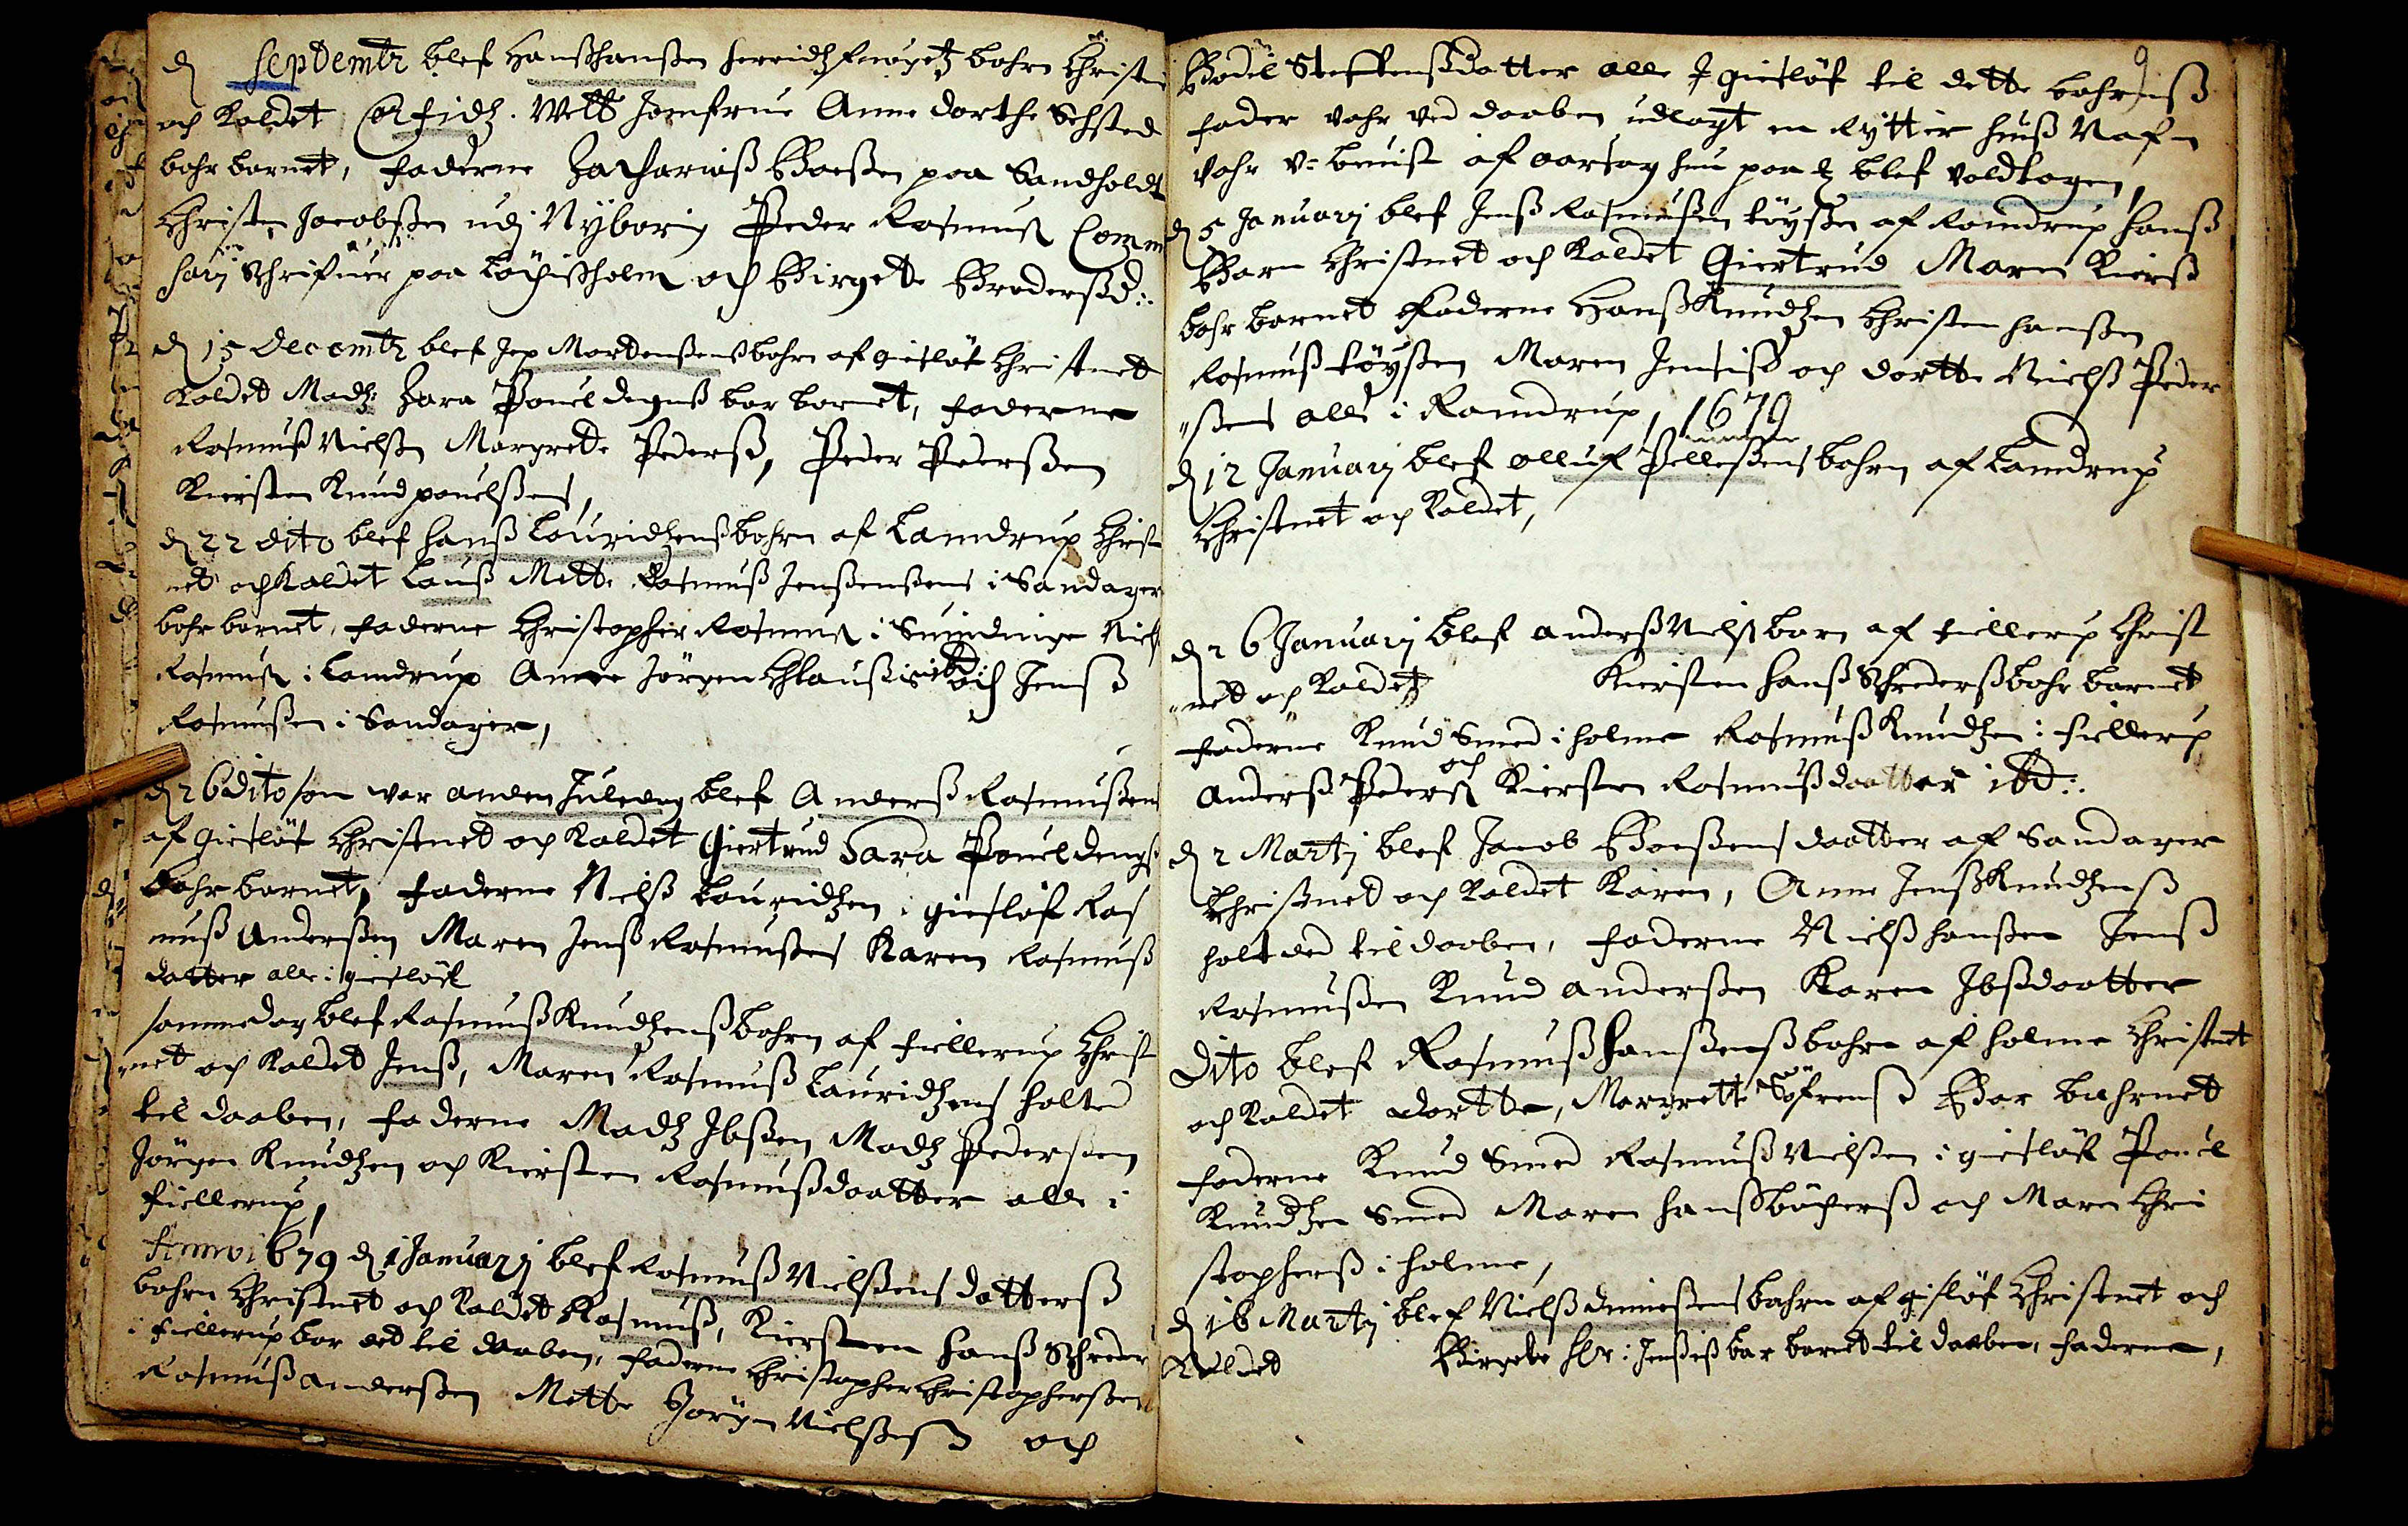d Septembr blef Hanss Hanssen Herridtz fuogetz bahrn Christn
och kaldet Corfidtz.  Well Jomfrue Anne Dorthe Sehsted
bahr barnet, Faderne Zachariass Boessen paa Sandholdt
Christen Jacobssen udi Nyborg Peder Rasmus Comm
harij## schrifuer paa Løchissholm och Birgette Brodersd:.
d 15 Decemtr blef Jep Mortenssenss bahrn af giesløf Christnet
kaldet Madtz Zara Pouel degnss bar barnet, Faderne
Rasmusss Nielss Margrete Pederss, Peder Pederssen
Kiersten Knud pouelssens
d 22 dito blef Hanss Louridtzenss bahrn af Lamdrup Christ¬
net och kaldet Lauss Mette Rasmuss Jenssenssenss i Sandager
bahr barnet, Faderne Christopher Rasmuss i Suindinge Niels 
Rasmuss i Lamdrup Anne Jørgen Claussis ibd: Jenss
Rasmussen i Sandager,
d 26 dito som var anden Juledag blef Anders Rasmussens
af Giesløf Christnet og kaldet Giertrud Sara Pouel Dengs
bahr barnet, Faderne Nielss Lauridtzen i giesløf Ras
muss Anderssen Maren Jenss Rasmussens Karen Rasmuss
datter alie i giesløf
samme dag blef Rasmuss Knudtzenss bahrn af fiellerup Christ¬
= net och kaldet Jenss, Maren Rasmuss Lauridtzens holte
til daaben, Faderne Madtz Ibssen Madtz Pederssen
Jørgen Knudtzen och Kiersten Rasmussdaatter alle i
Fiellerup
Anno 1679 d 1 Jaruarij blef Rasmuss Nielssens datterss
bahrn Christnet og kaldet Rasmuss Kiersten Hanss Schreder
i Fiellerup bar det til Daaben, Faderene Christopher Christophersen
Rasmuss Anderssen Mette Jørgen Nielssess och
9.
Bodil Steffenssdatter alle I Giesløf til dette bahrnss
fader vahr ved daaben udlagt en Rytter huess Nafn
vohr v=beuist af aarsag hun paa ## blef voldagen
d 5 Januarij blef Jenss Rasmussen tøyssen af Ramdrup Hanss
Barn Christnet och kaldet Giertrud Maren Kierss
bahr barnet Faderne Hanss Knudtzen Christen Hanssen
Rasmuss tøyssen Maren Jensiss och Dortte Nielss Peder
= sens alle i Ramdrup, 1679
D 12 Januarj bleff Olluff Pellersens bahrn af Lauedrup
Christnet og kaldet,
d 26 Januarij bleff Anderss Niels barn af Fiellerp Christ
= net och kaldet
Kiersten Hanss Schrederss bahr barnet
Faderne Knud Smed i Holme Rasmuss Knudtzen i Fiellerup
och
Anderss Peders Kiersten Rasmusdaatter ibd:.
d 2 Martij blef Jacob Boessens daatter af Sandager
Christned och kaldet Karen, Anne Jenss Knudtzenss
holt det til daaben, Faderne Nielss Hanssen, Jenss
Rasmssen Knud Anderssen Karen Ibssdaatter
Dito blef Rasmuss Hanssenss bahrn af Holme Christenet
och kaldet Dortte, Margrette Søfrenss Bar bahrnet
Faderne Knud Smed Rasmuss Nielssen i giesløf Pouel
Knudtzen Smed Maren Hanss bøcherss och Maren Chri
stopherss i holme
d 16 Martij blef Nielss dinnessens bahrn af gisløf Christenet och
kaldet
Birgete hlr: Jenssess bar barnet til Daaben, Faderne,
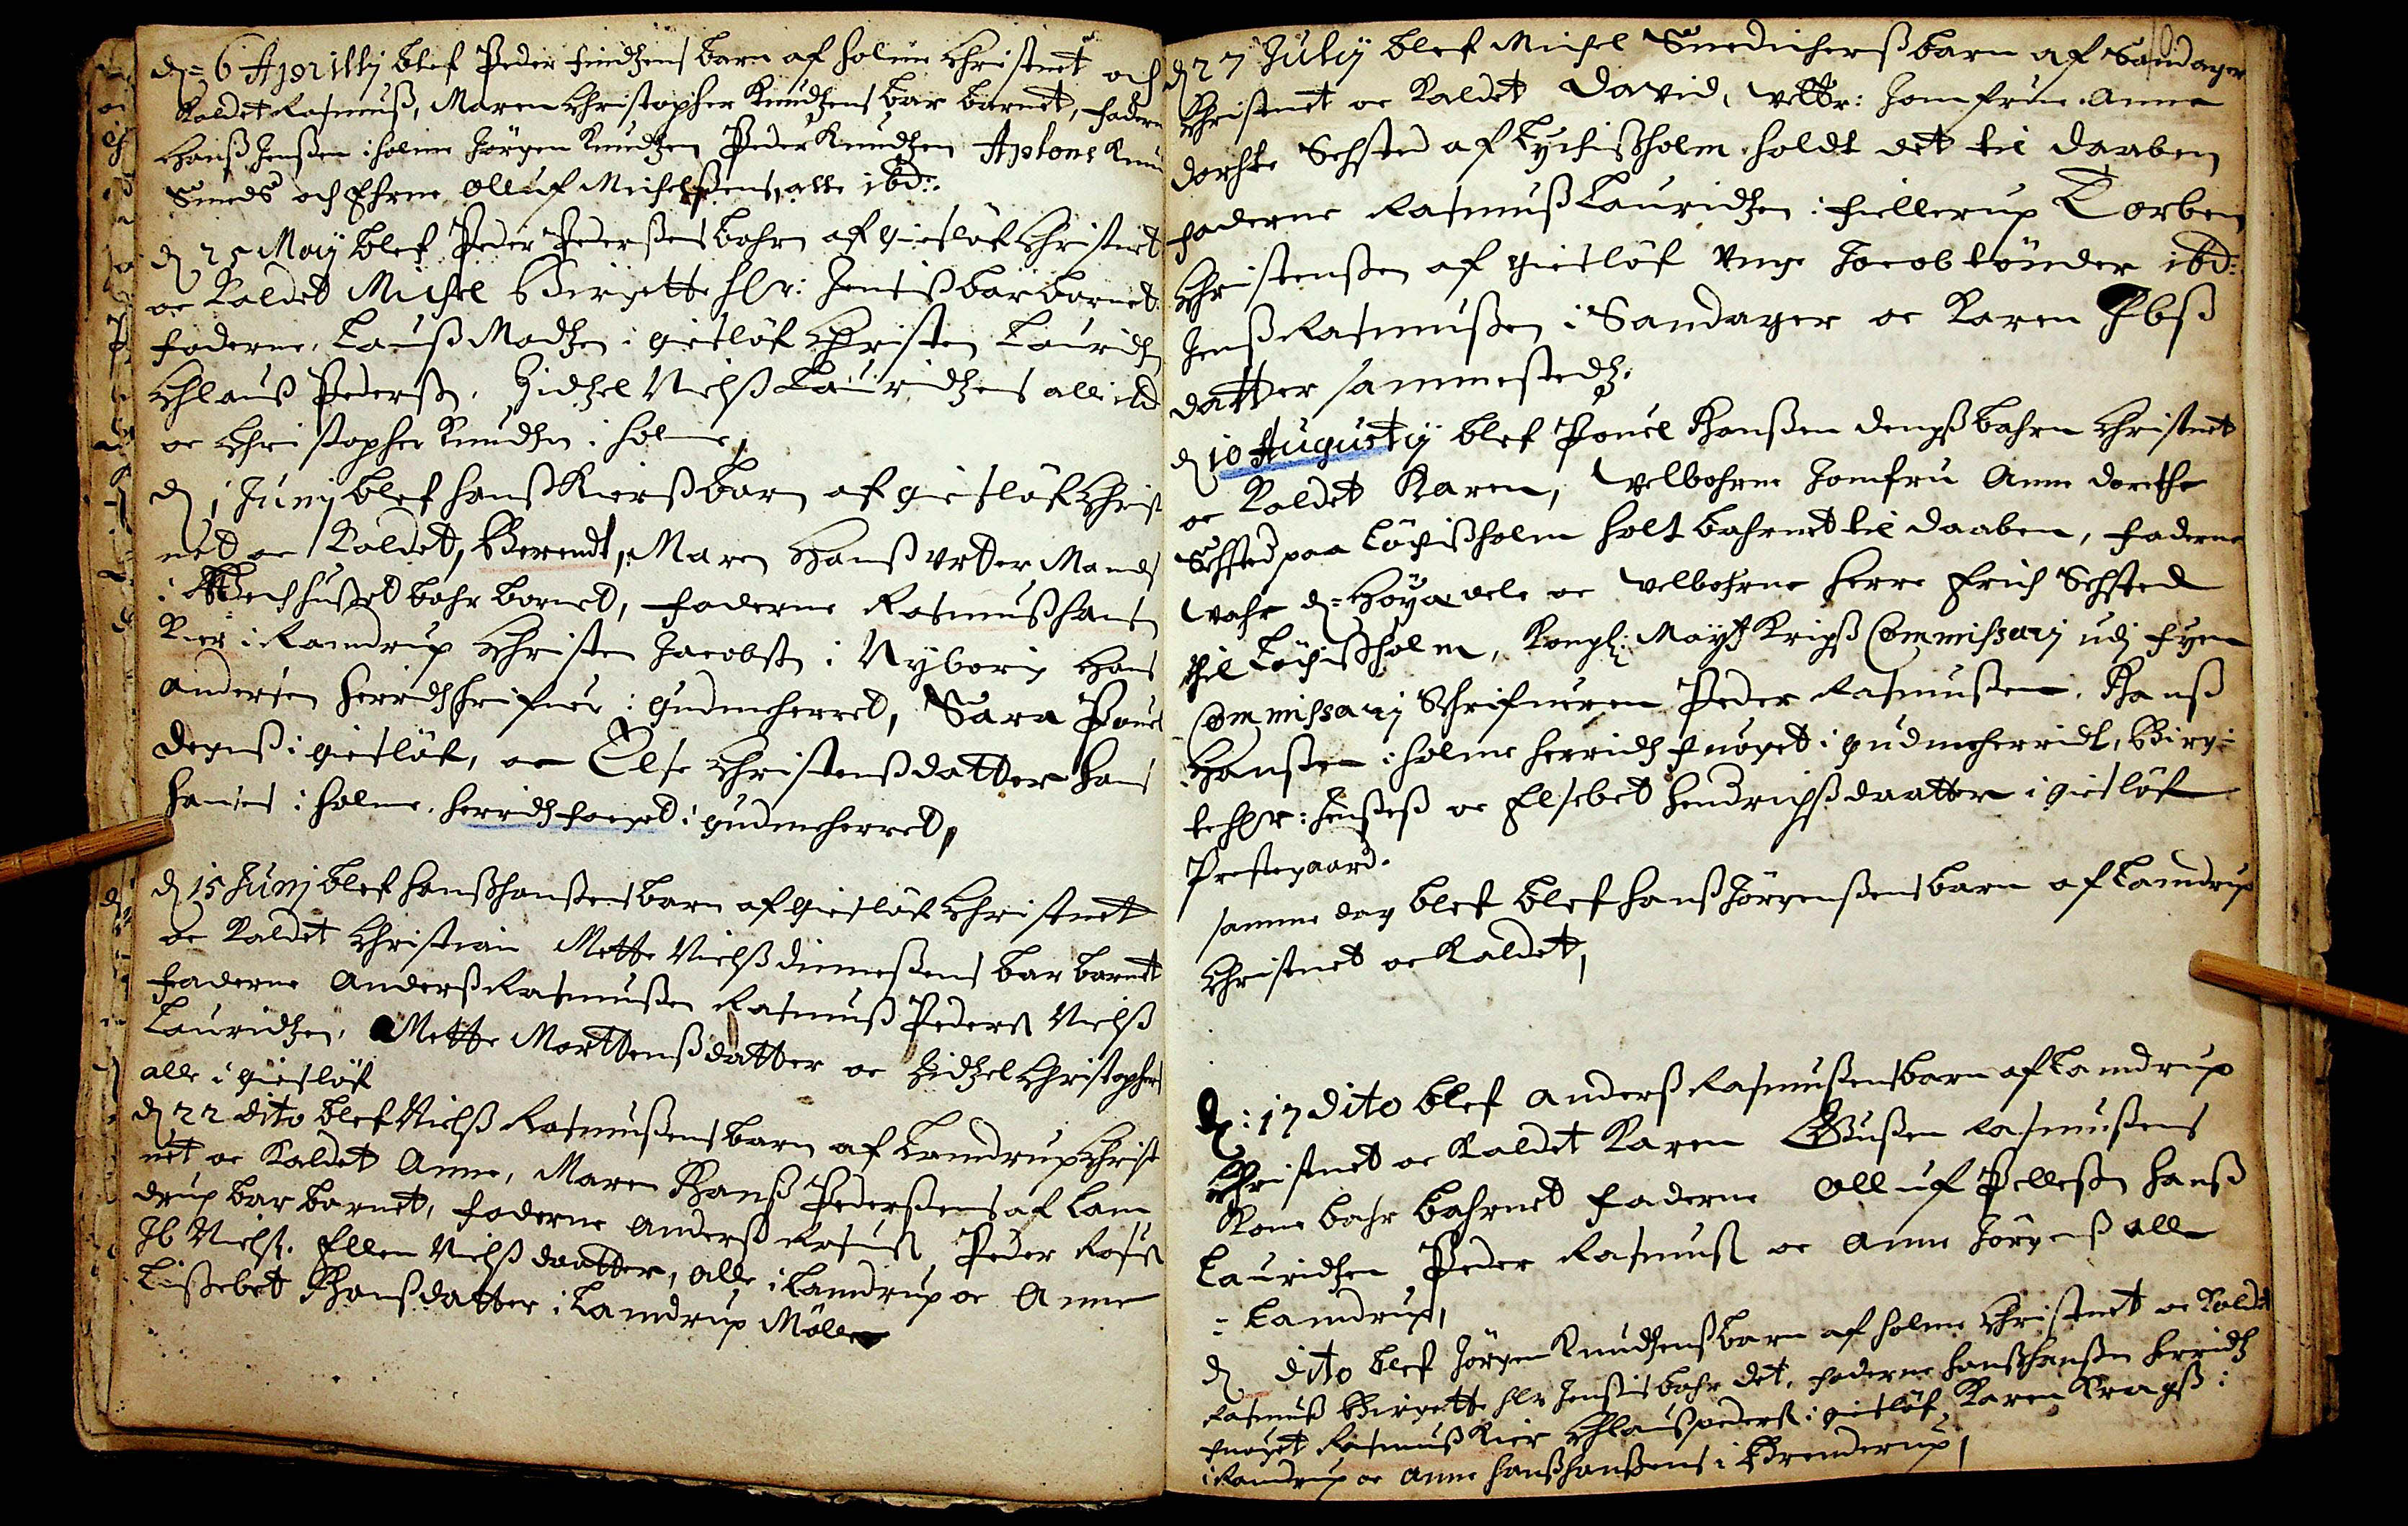d= 6 Aprillij blef Peder Findzens barn af Holme Christnet och
kaldet Rasmuss, Maren Christopher Knudtzens bar barnet, Faderne
Hanss Jenssen i Holme Jørgen Knudtzen Peder Knudtzen i Apolone
Smeds och ##hrne Olluf Michelssens, alle ibd:.
d 25 Maij blef Peder Pederssens bahrn af Giesløf Christenet
oc kaldet Michel Birgette Hlr: Jensiss bar barnet
Faderne, Lauss Madtzen i Giesløf Christen Lauridtz
Chlaus Pederss. Zidtzel Nielss Lauridtzens alle ibd
oc Christopher Knudtzen i Holme,
d 1 Junij blef Hanss Kierss barn af Giesløf Christ
net oc Kaldet, Berendt, Maren Hanss vrter Mands
i Bechhuset bahr barnet, Faderne Rasmuss Hansen
Kier i Ramdrup Christen Jacobss i Nyborg Hans
Andersen Herridtz## i Gudmeherret, Sara Pouel
Degns i Giesløf, oc Else Christenssdatter Hans
Hansens i Holme. Herridtzfoeget i Gudmeherret,
d 15 Junij blef Hanss Hanssens barn af Giesløf Christnet
oc kaldet Christran Mette Nielss Dienessens barn barnet
Faderne Anderss Rasmussen Rasmuss Peders Niels
Lauridtzen, Mette Morttenssdatter oc Zidtzel Christophers
alle i Giesløf
d 22 dito blef Nielss Rasmussens barn af Lamdrup Christ
net oc kaldet Anne, Maren Hans Pederssens af Lam
drup bar barnet, Faderne Anderss Rasmuss, Peder
Ib Nielss. Ellen Nielssdaatter, alle i Lamdrup oc Anne
Lissebet Hansdatter i Lamdrup Mølle
10.
d 27 Julij blef Michel Snedicherss barn af Sandager
Christenet oc kaldet David, Velbr: Jomfrue Anne
Dorthe Sehsted af Lychissholm holdt det til daaben
Faderne Rasmuss Lauridtzen i Fiellerup Torben
Christenssen af Giesløf vnge Jacobo tønder ibd:
Jens Rasmssen i Sandager oc Karen Ibss
datter sammestedtz.
d 10 Augustij blef Pouel Hanssen Degnss bahrn Christenet
opc kaldet Karen, Velbohrne Jomfru Anne Dorethe
Sehsted paa Løchissholm holt bahrnet til Daaben, Faderne
vahr d=Høya## oc velbohrne Herre Frich Sehsted
til Løchissholm, Kongl: Mayt Krigss Commissarij udi Fyen
Commissarij Schrifueren Peder Rasmussen, Hanss
Hansen i Holme Herridtzfuoget i Gudmeherridt, Birgi
te hlr: Jensess oc Elsebet Hendrichssdaatter i Giesløf
Prestegaard.
samme dag blef blef Hanss Jørgenssens barn af Lamdrup
Christnet oc kaldet,
d: 17 dito blef Anderss Rasmussens barn af Lamdrup
Christnet oc kaldet Karen ##Bussen Rasmussens
Kone bahr bahrnet Faderne Olluf Pellessen Hanss
Lauridtzøn Peder Rasmus oc Anne Jørgenss alle
i Lamdrup
d dito blef Jørgen Knudtzens barn af Holme Christenet, oc kaldet
Rasmuss Birgette hlr Jenssis bafr det. Faderne Hans Hansen Herridtz
fuoget Resmuss Kier Chlaus peders i Gisløf Karen Krags:
i Ramdrup oc Anne Hans Hanssens i Brenderup,